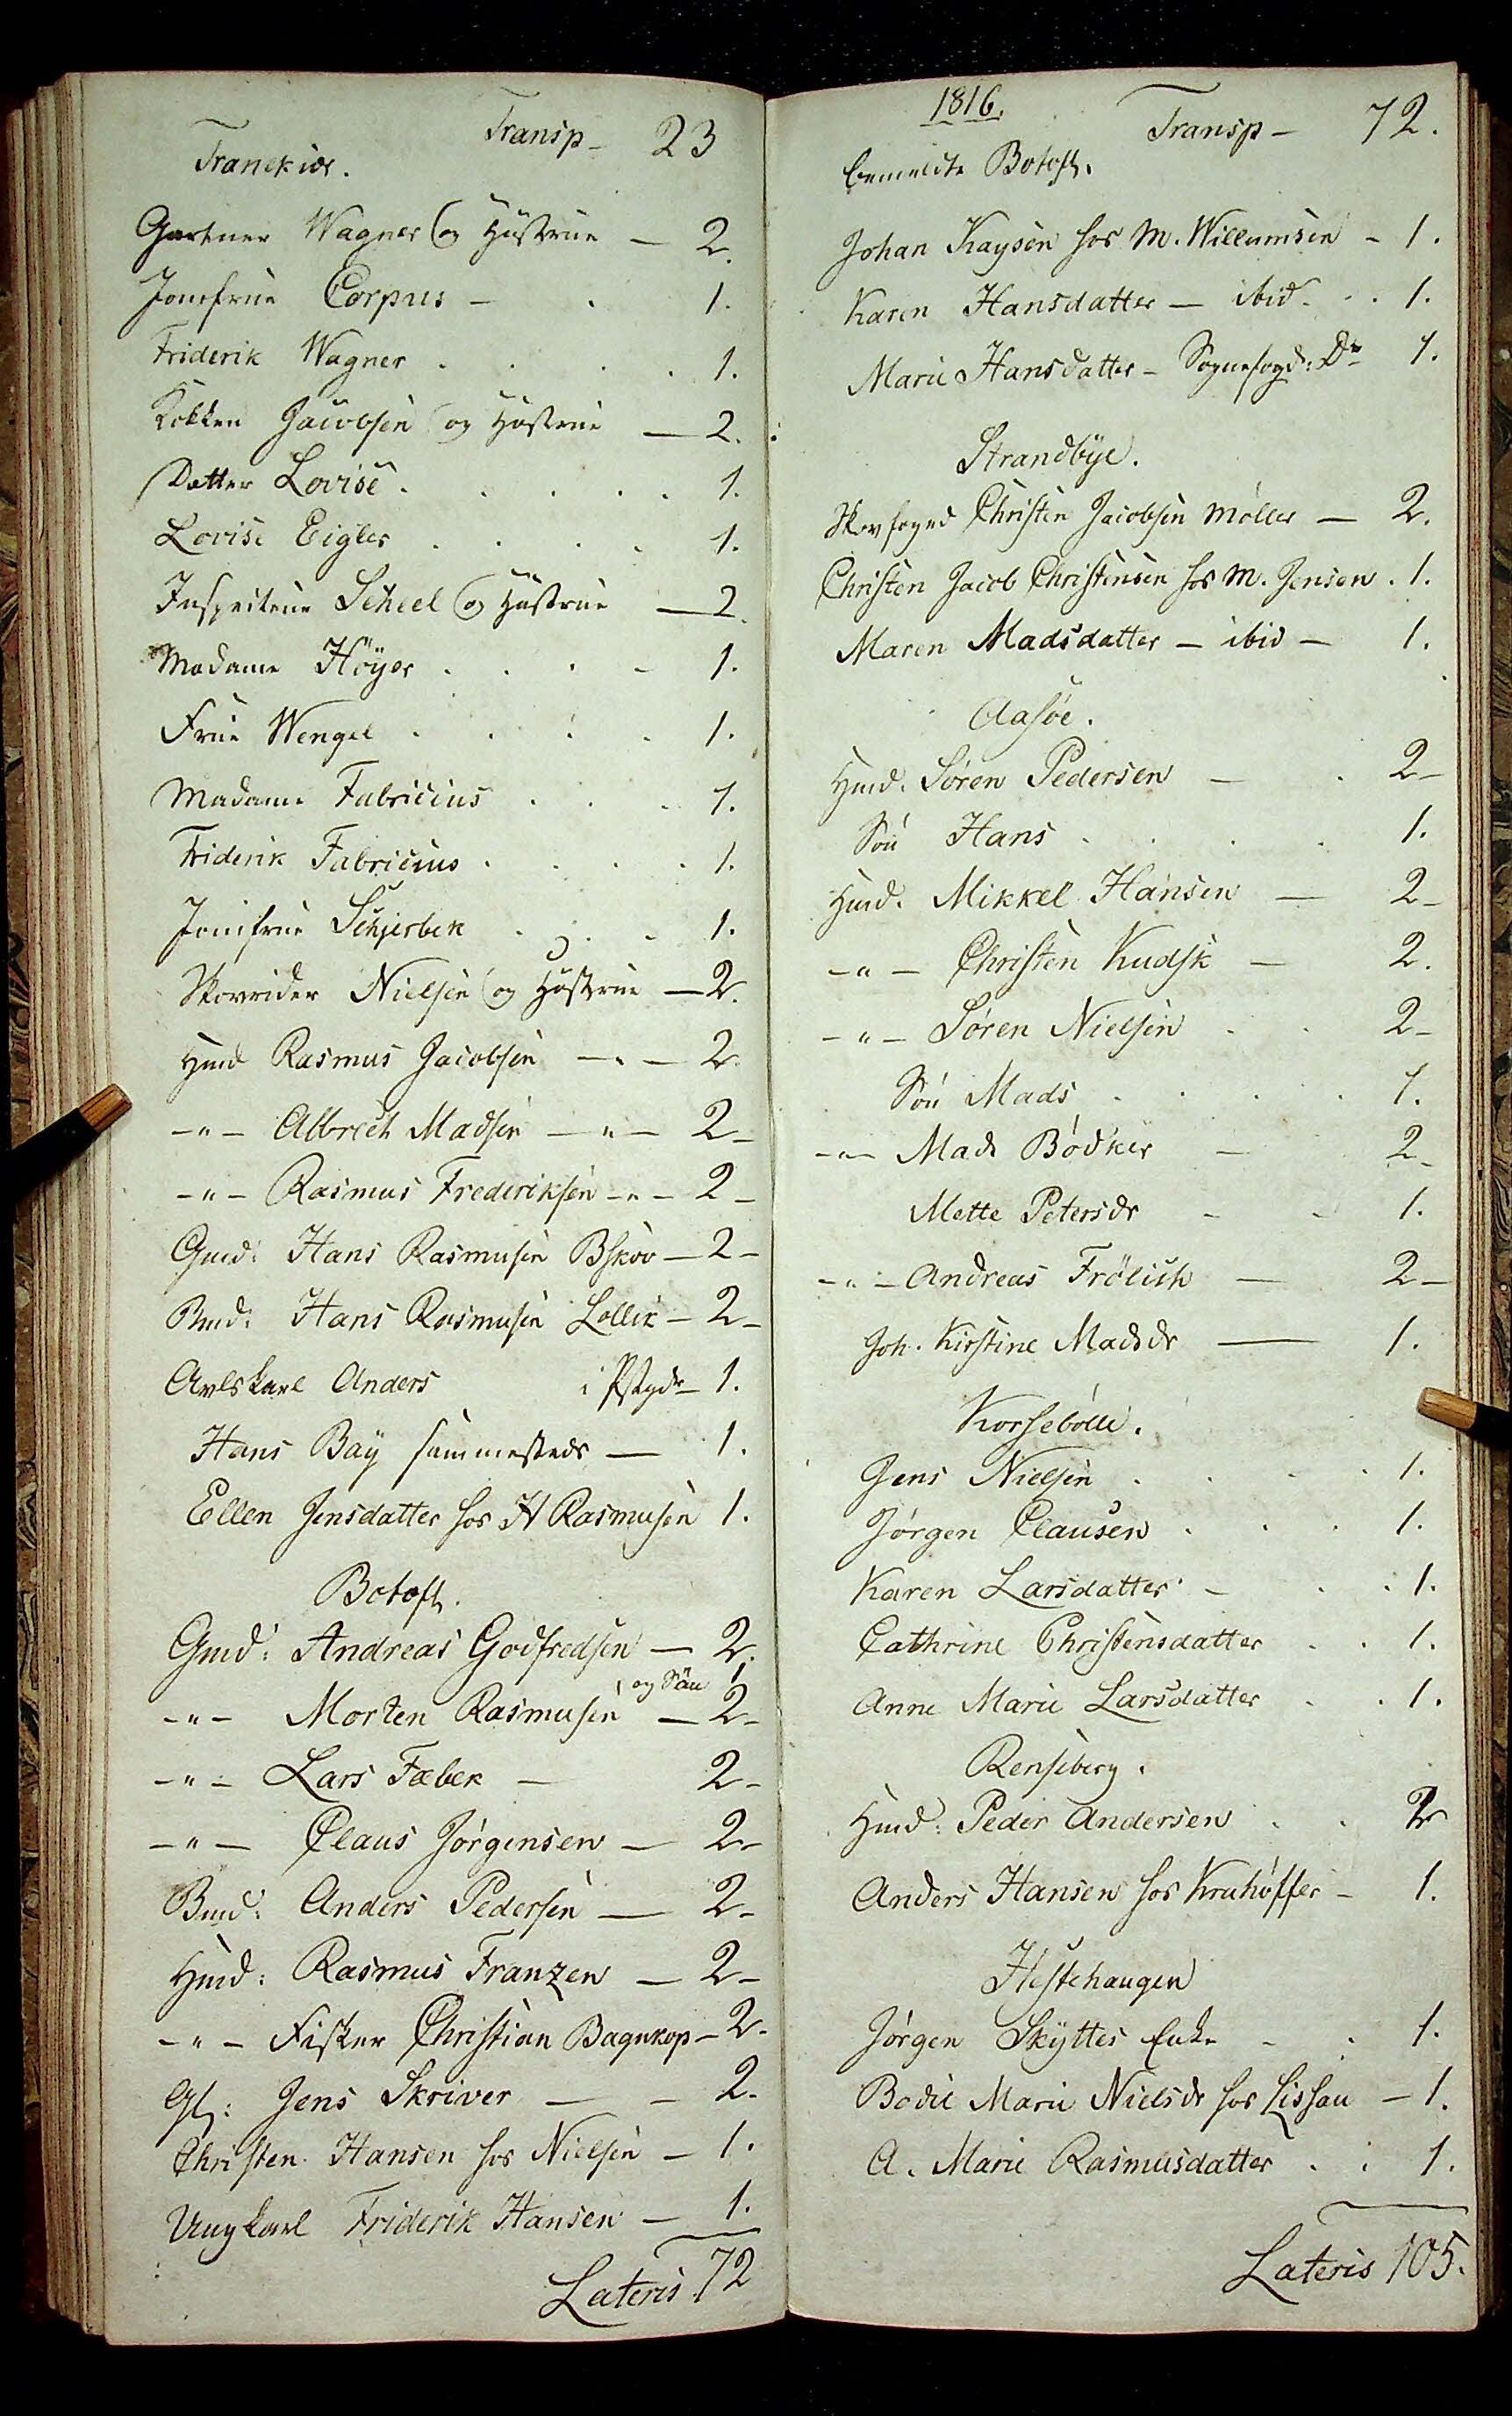Transp - 23
Tranekiær.
Gartner Wagner og Hustrue - 2
Jomfrue Corpus - . 1.
Friderik Wagner . . . . 1.
Kokken Jacobsen og Hustrue - 2.
Datter Lovise . . . . . 1.
Lovise Eigler . . . . 1.
Inspecteur Scheel og Hustrue - 2
Madame Høyer . . . . 1.
Frue Wengel . . . . 1.
Madame Fabricius . . . 1.
Friderik Fabricius . . . . 1.
Jomfrue Schjerbek . . . 1.
Skovrider Nielsen og Hustrue - 2.
Hmd Rasmus Jacobsen -"- 2.
-"- Albrect Madsen -"- 2-
-"- Rasmus Frederiksen -"- 2-
Gmd: Hans Rasmusen Bskov - 2-
Bmd: Hans Rasmusen Lollik - 2-
Avlskarl Anders i Pstgdr 1.
Hans Bay sammesteds - 1.
Ellen Jensdatter hos H Rasmusen 1.
Botoft
Gmd: Andreas Godfredsen - 2.
og Søn 1
-"- Morten Rasmusen - 2-
-"- Lars Fæbek - 2-
-"- Claus Jørgensen - 2-
Bmd: Anders Pedersen - 2-
Hmd: Rasmus Franzen - 2-
-"- Fisker Christian Bagnkop - 2.
gl: Jens Skriver - - 2.
Christen Hansen hos Nielsen - 1.
Ungkarl Friderik Hansen - 1.
Lateris 72
1816.
Transp - 72.
bemeldte Botoft.
Johan Kaysen hos M: Willumsen - 1.
Karen Hansdatter - ibid . . . 1.
Marie Hansdatter - Sognefogd: Dr 1.
Strandbye.
Skofoged Christen Jacobsen Møller- 2.
Christen Jacob Christensen hos M. Jensen . 1.
Maren Madsdatter - ibid - 1.
Aasøe.
Hmd. Søren Pedersen - . 2-
Søn Hans . . . . 1.
Hmd. Mikkel Hansen - 2-
-"- Christen Kudsk - 2.
-"- Søren Nielsen . .2-
Søn Mads . . . . 1.
-"- Mads Bødker - 2-
Mette Petersdr - - 1.
-"- Andreas Frølich - 2-
Joh. Kirstine Madsdr - 1.
Korsebølle.
Jens Nielsen . . . . 1.
Jørgen Clausen . . . 1.
Karen Larsdatter - . . 1.
Cathrine Christensdatter . . 1.
Anne Marie Larsdatter . . 1.
Renseberg.
Hmd: Peder Andersen . . 2
Anders Hansen hos Kruhøffer - 1.
Hestehaugen
Jørgen Skyttes Enke - . 1.
Bodil Marie Nielsdr hos Lissau - 1.
A. Marie Rasmusdatter . . 1.
Lateris 105.
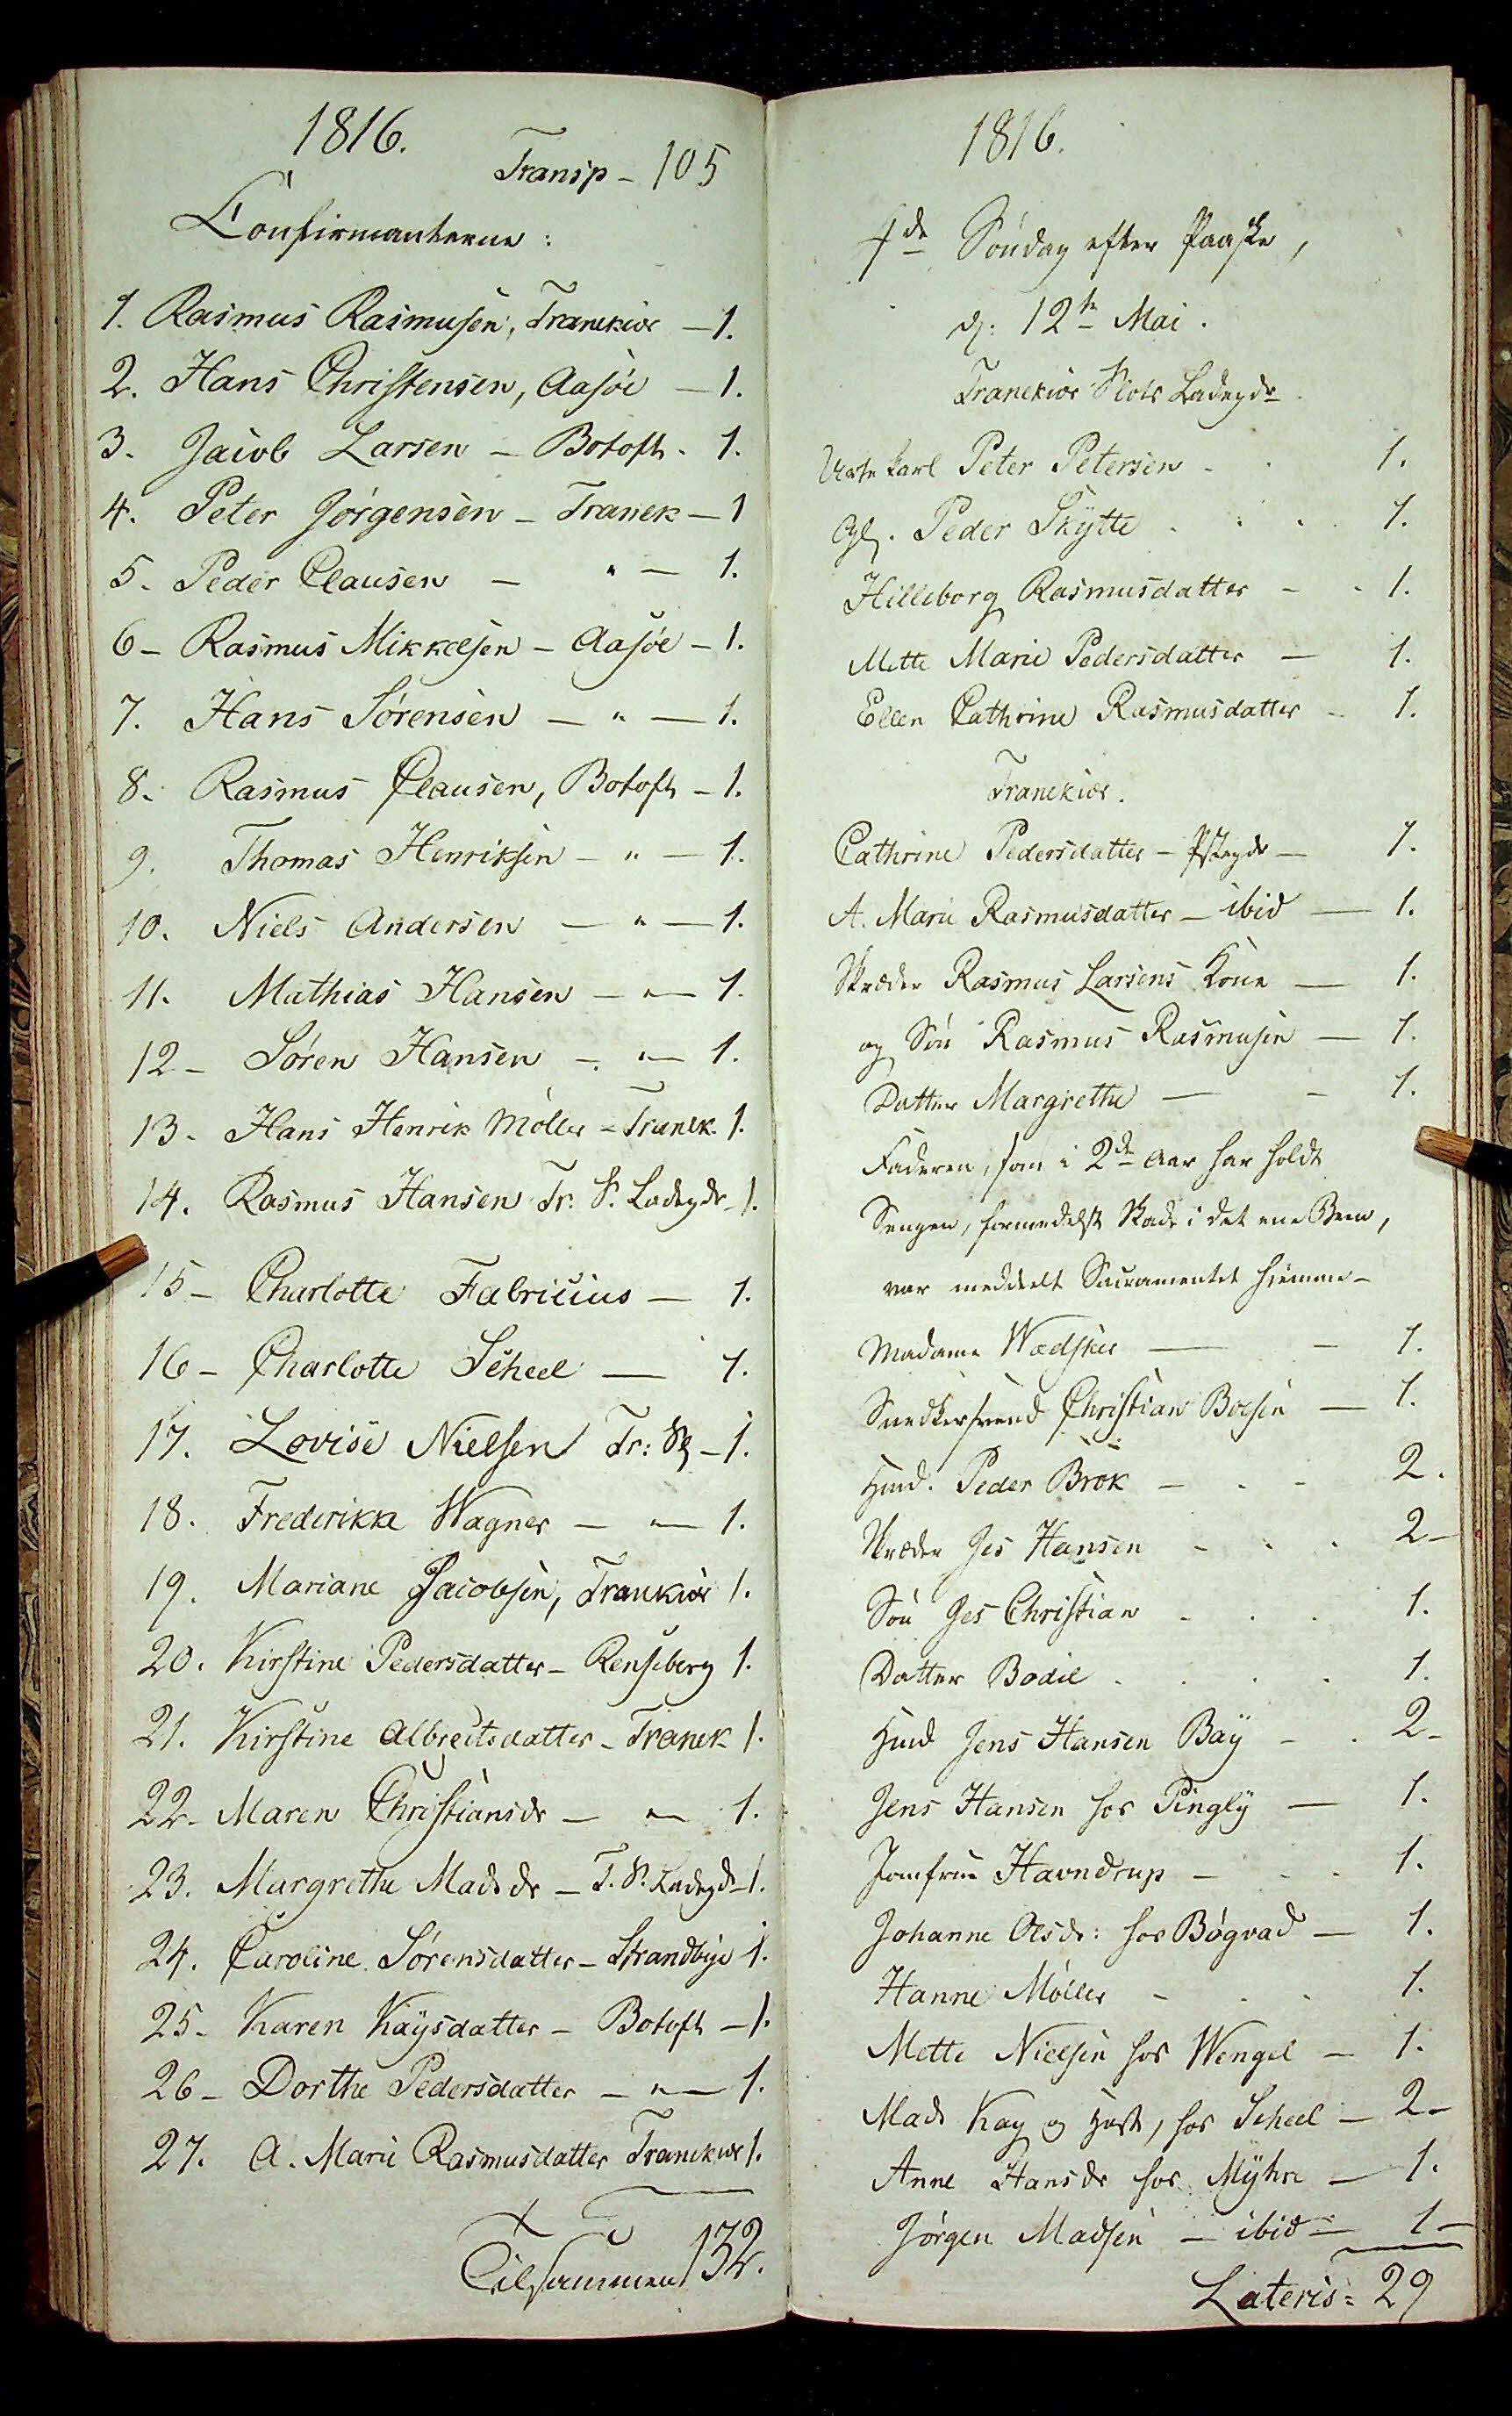1816.
Transp - 105
Confirmanterne:
1. Rasmus Rasmusen, Tranekiær - 1.
2. Hans Christensen, Aasøe - 1.
3. Jacob Larsen - Botoft . 1.
4. Peter Jørgensen Tranek . 1.
5. Peder Clausen -"- 1.
6 - Rasmus Mikkelsen - Aasøe 1.
7. Hans Sørensen -"- 1.
8. Rasmus Clausen, Botoft - 1.
9, Thomas Henriksen -"- 1.
10. Niels Andersen -"- 1.
11. Mathias Hansen -"- 1.
12 - Søren Hansen - "- 1.
13. Hans Henrik Møller - Tranek . 1.
14. Rasmus Hansens Tr: S. Ladegdr 1.
15 - Charlotte Farbricius - 1.
16 - Charlotte Scheel - 1.
17. Lovise Nielsen Tr: Sl -1.
18. Frederikke Wagner - - 1.
19. Mariane Jacobsen, Tranekiær 1.
20. Kirstin Pedersdatter - Renseberg 1.
21. Kirstine Albretsdatter - Tranek 1.
22. Maren Christiansdr - - 1.
23. Margrethe Madsdr - T. S. Ladegd - 1.
24. Caroline Sørensdatter - Strandbye 1.
25. Karen Kaysdatter - Botoft - 1
26. Dorthe Pedersdatter -"- 1
27. A. Marie Rasmusdatter Tra<nekiær 1.
Tilsamme 132.
1816.
4de Søndag efter Paaske
d: 12te Mai.
Tranekiær Slots Ladegdr
Urtekarl Peter Petersen . . 1.
gl. Peder Skytte . . . 1.
Hilleborg Rasmusdatter - . 1.
Mette Marie Pedersdatter - 1.
Ellen Cathrine Rasmusdatter - 1.
Tranekiær.
Cathrine Pedersdatter - Pstgdr - 1.
A. Marie Rasmusdatter - ibid - 1.
Skræder Rasmus Larsens Kone - 1.
og Søn Rasmus Rasmusen - 1.
Datter Margrethe - - 1.
Faderen, som i 2de Aar har holdt
Sengen, formedet Skade i det en Been,
var meddelt Sacramentet hiemme -
Madam Wædsker - - 1.
Snedkersvend Christian Boesen - 1.
Hmd. Peder Brok - - - 2
Skræder Jes Hansen -  . . 2
Søn Jes Christian . . . 1.
Datter Bodil . . . . 1.
Hmd Jens Hansen Bay - . 2-
Jens Hansen hos Pingly - 1.
Jomfrue Havndrup - . . 1.
Johanne Olsdr: hos Bøgvad - 1.
Hanne Møller - . . 1.
Mette Nielsen hos Wengel - 1.
Mads Kay og Hust, hos Scheel - 2-
Anne Hansdr hos Myhre - 1.
Jørgen Madsen - ibid - 1
Latteris = 29